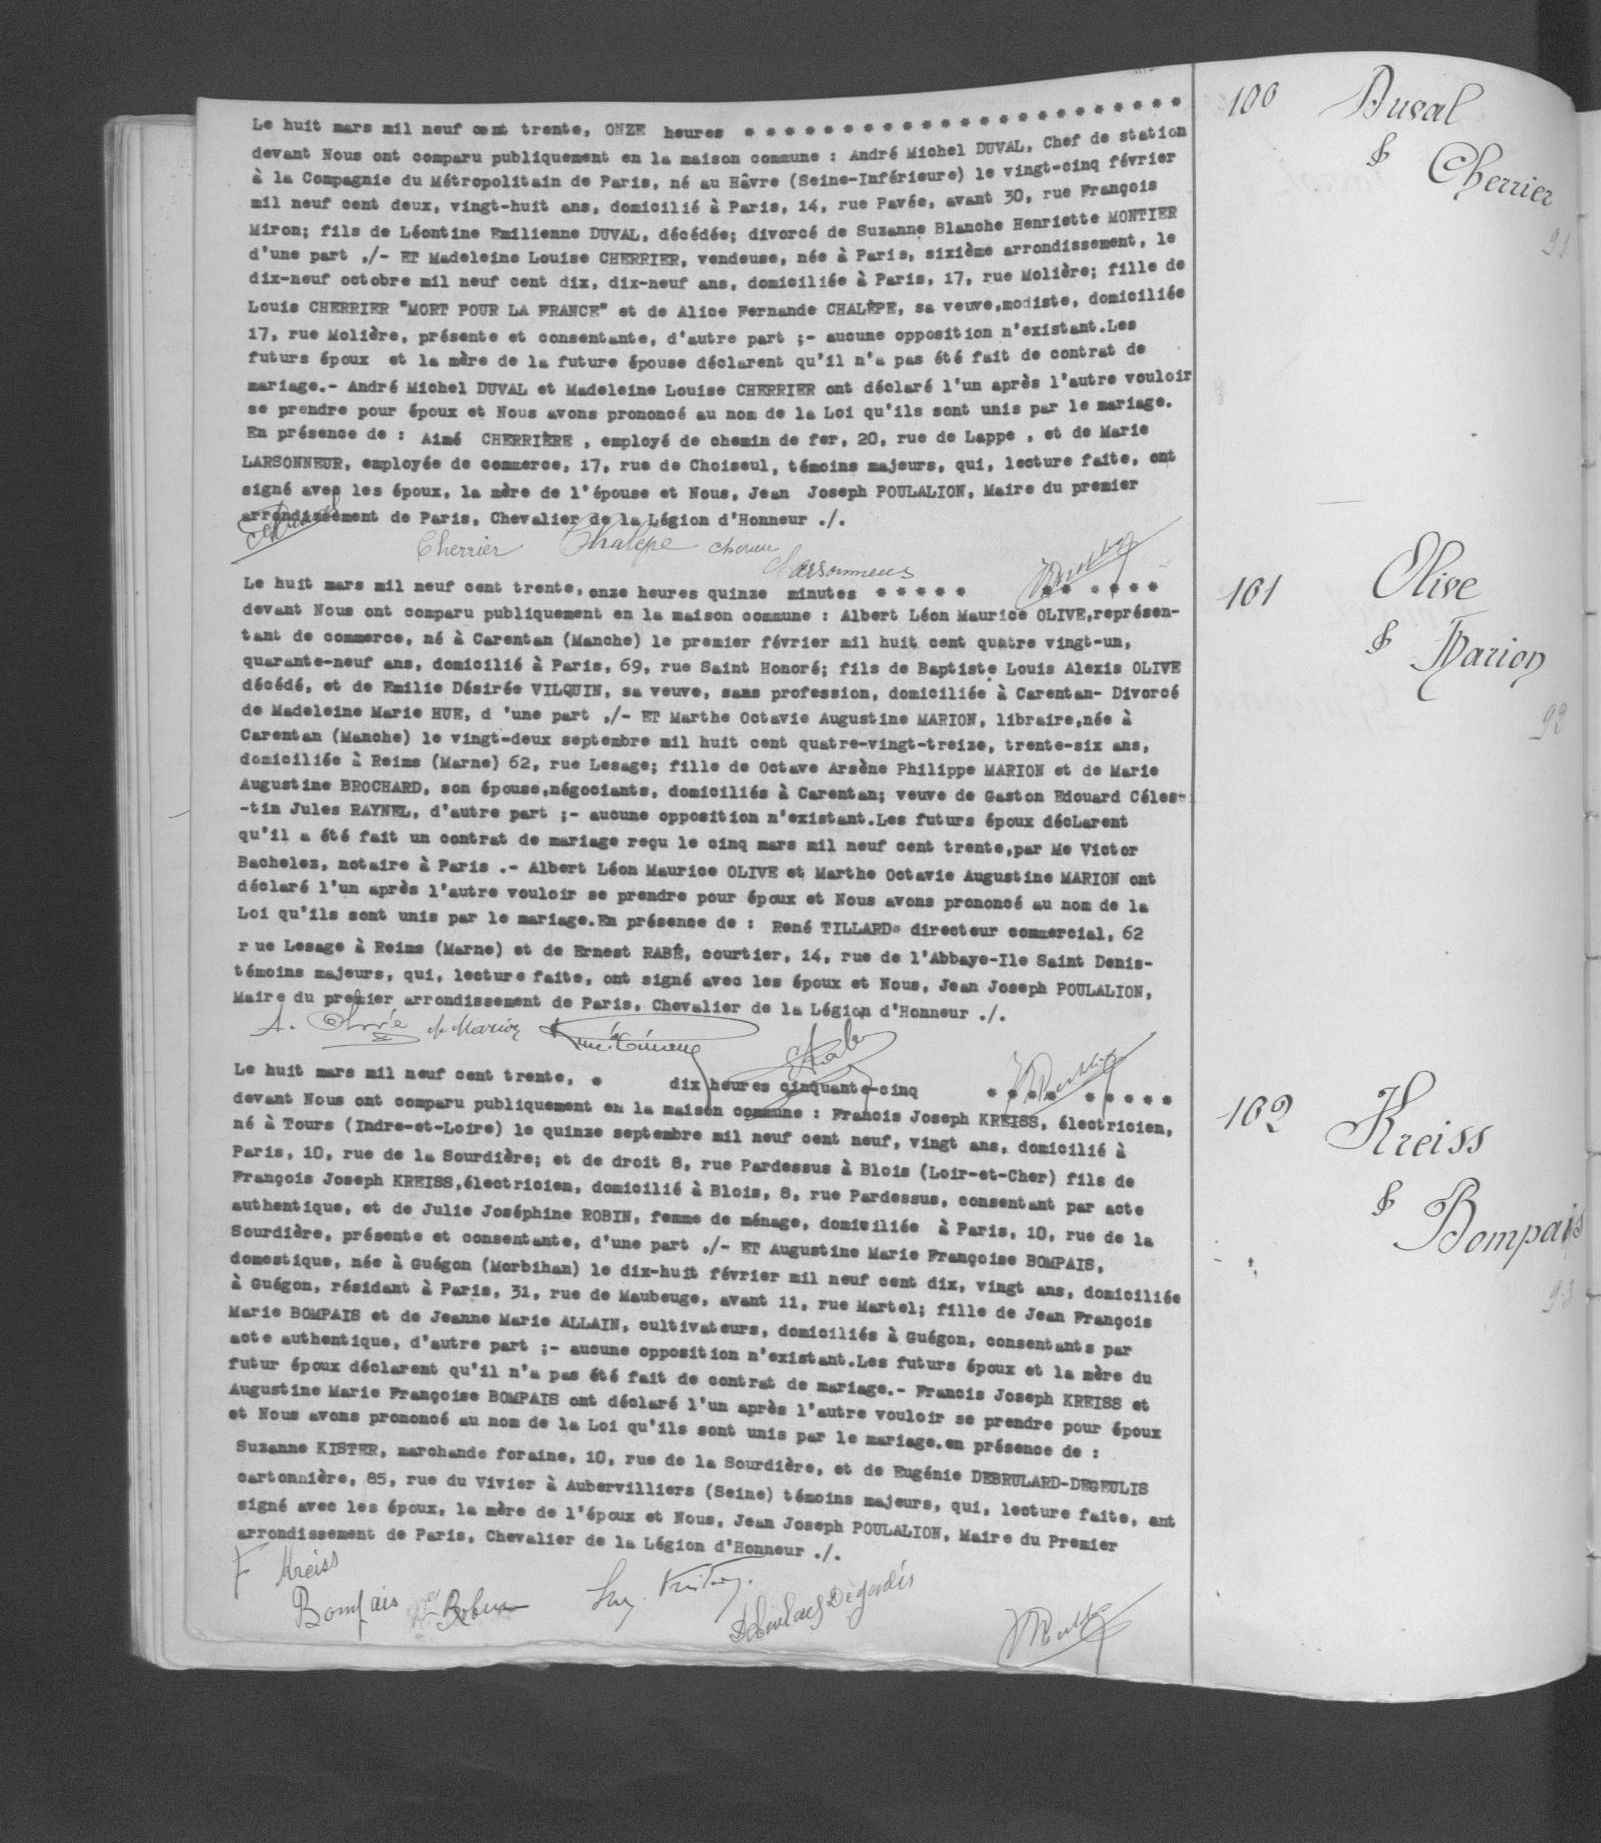Le huit mars mil neuf cent trente, onze heures ****
devant Nous ont comparu publiquement en la maison commune: André Michel DUVAL Chef de station
à la Compagnie du Métropolitain de Paris, né au Hâvre (Seine-Inférieure) le vingt-cinq février
mil neuf cent deux, vingt-huit ans, domicilié à Paris, 14, rue Pavée, avant 30, rue François
Miron; fils de Léontine Emilienne DUVAL, décédée; divorcé de Suzanne Blanche Henriette MONTIER
d'une part ,/-ET Madeleine Louise CHERRIER, vendeuse, née à Paris, sixième arronidssement, le
dix-neuf octobre mil neuf cent dix, dix-neuf ans, domiciliée à Paris, 17, rue Molière; fille de
Louis CHERRIER "MORT POUR LA FRANCE" et de Alice Fernande CHALEPE, sa veuve, modiste, domiciliée
17, rue Molière, présente et consentante, d'autre part ;-aucune opposition n'existant.Les
futurs époux et la mère de la future épouse déclarent qu'il n'a pas été fait de contrat de
mariage .-André michel DUVAL et Madeleie Louise CHERRIER ont déclaré l'un après l'autre vouloir
se prendre pour époux et Nous avons prononcé au nom de la Loi qu'ils sont unis par le mariage.
En présence de: Aimé CHERRIERE, employé de chemin de fer, 20, rue de Lappe, et de Marie
LARSONNEUR, employée de commerce, 17, rue de Choiseul, témoins majeurs, qui lecture faite, ont
signé avec les époux, la mère de l'épouse et Nous, Jean Joseph POULALION, Maire du premier
arrondissement de Paris, Chevalier de la Légion d'Honneur ./.
Le huit mars mil neuf cent trente, onze heures quinze ****
devant Nous ont comparu publiquement en la maison commune: Albert Léon Maurice OLIVE, représen-
tant de commerce, né à Carentan (Manche) le premier février mil huit cent quatre vingt-un,
quarante-neuf ans, domicilié à Paris, 69, rue Saint Honoré; fils de Baptiste Louis Alexis OLIVE
décédé, et de Emilie Désirée VILQUIN, sa veuve, sans profession, domiciliée à Carentan-Divorcé
de Madeleine Marie HUE, d'une part ,/-ET Marthe Octavie Augustine MARION, libraire, née à
Carentan (Manche) le vingt-deux septembre mil huit cent quatre-vigt-treize, trente-six ans,
domiciliée à REims (Marne) 62, rue Lesage; fille de Octave Arsène Philippe MARION et de Marie
Augustine BROCHARD, son épouse, négociante, domiciliés à Carentan; veuve de Gaston Edouard Céles-
-tin Jules RAYNEL, d'autre part ;-aucune opposition n'existant. Les futurs époux décLarent
qu'il a été fait un contrat de mariage reçu le cinq mars mil neuf cent trente, par Me Victor
Bachelez, notaire à Paris .-Albert Léon Maurice OLIVE et Marthe Octavie Augustine MARION ont
déclaré l'un après l'autre vouloir se prendre pour époux et Nous avons prononcé au nom de la
Loi qu'ils sont unis par le mariage. En présence de: René TILLARD * directeur commercial, 62
rue Lesage à Reims (Marne) et de Ernest RABE, courtier, 14, rue de l'Abbaye-Ile Saint Denis-
témoins majeurs, qui, lecture faite, ont signé avec les époux et Nous, Jean Joseph POULALION,
Maire du premier arrondissement de Paris, Chevalier de la Légion d'Honneur ./.
Le huit mars mil neuf cent trente, * dix heures cinquante-cinq ****
devant Nous ont comparu publiquement en la maison commune: Francis Joseph KREISS, électricien,
né à Tours (Indre-et-Loire) le quinze septembre mil neuf cent neuf, vingt ans, domicilié à
Paris, 10, rue de la Sourdière; et de droit 8, rue Pardessus à Blois (Loir-et-Cher) fils de
François Joseph KREISS, électricien, domicilié à Blois, 8, rue Pardessus, consentant par acte
authentique, et de Julie Joséphine ROBIN, femme de ménage, domiciliée à Paris, 10, rue de la
Sourdière, présente et consentante, d'une part ,/-ET Augustine Marie Françoise BOMPAIS,
domestique, née à Guégon (Morbihan) le dix-huit février mil neuf cent dix, vingt ans, domiciliée
à Guégon, résidant à Paris, 31, rue de Maubeuge, avant 11, rue Martel; fille de Jean François
Marie BOMPAIS et de Jeanne Marie ALLAIN, cultivateurs, domiciliés à Guégon, consentants par
acte authentique, d'autre part ;-aucune opposition n'existant. Les futurs époux et la mère du
futur époux déclarent qu'il n'a pas été fait de contrat de mariage .-Francis Joseph KREISS et
Augustine Marie Françoise BOMPAIS ont déclaré l'un après l'autre vouloir se prendre pour époux
et Nous avons prononcé au nom de la Loi qu'ils sont unis par le mariage, en présence de:
Suzanne KISTER, marchande foraine, 10, rue de la Sourdière, et de Eugénie DEBRULARD-DEGEULIS
cartonnière, 85, rue du Vivier à Aubervilliers (Seine) témoins majeurs, qui, lecture faite, ont
signé avec les époux, la mère de l'époux et Nous, Jean Joseph POULALION, Maire du Premier
arrondissement de Paris, Chevalier de la Légion d'Honneur ./.
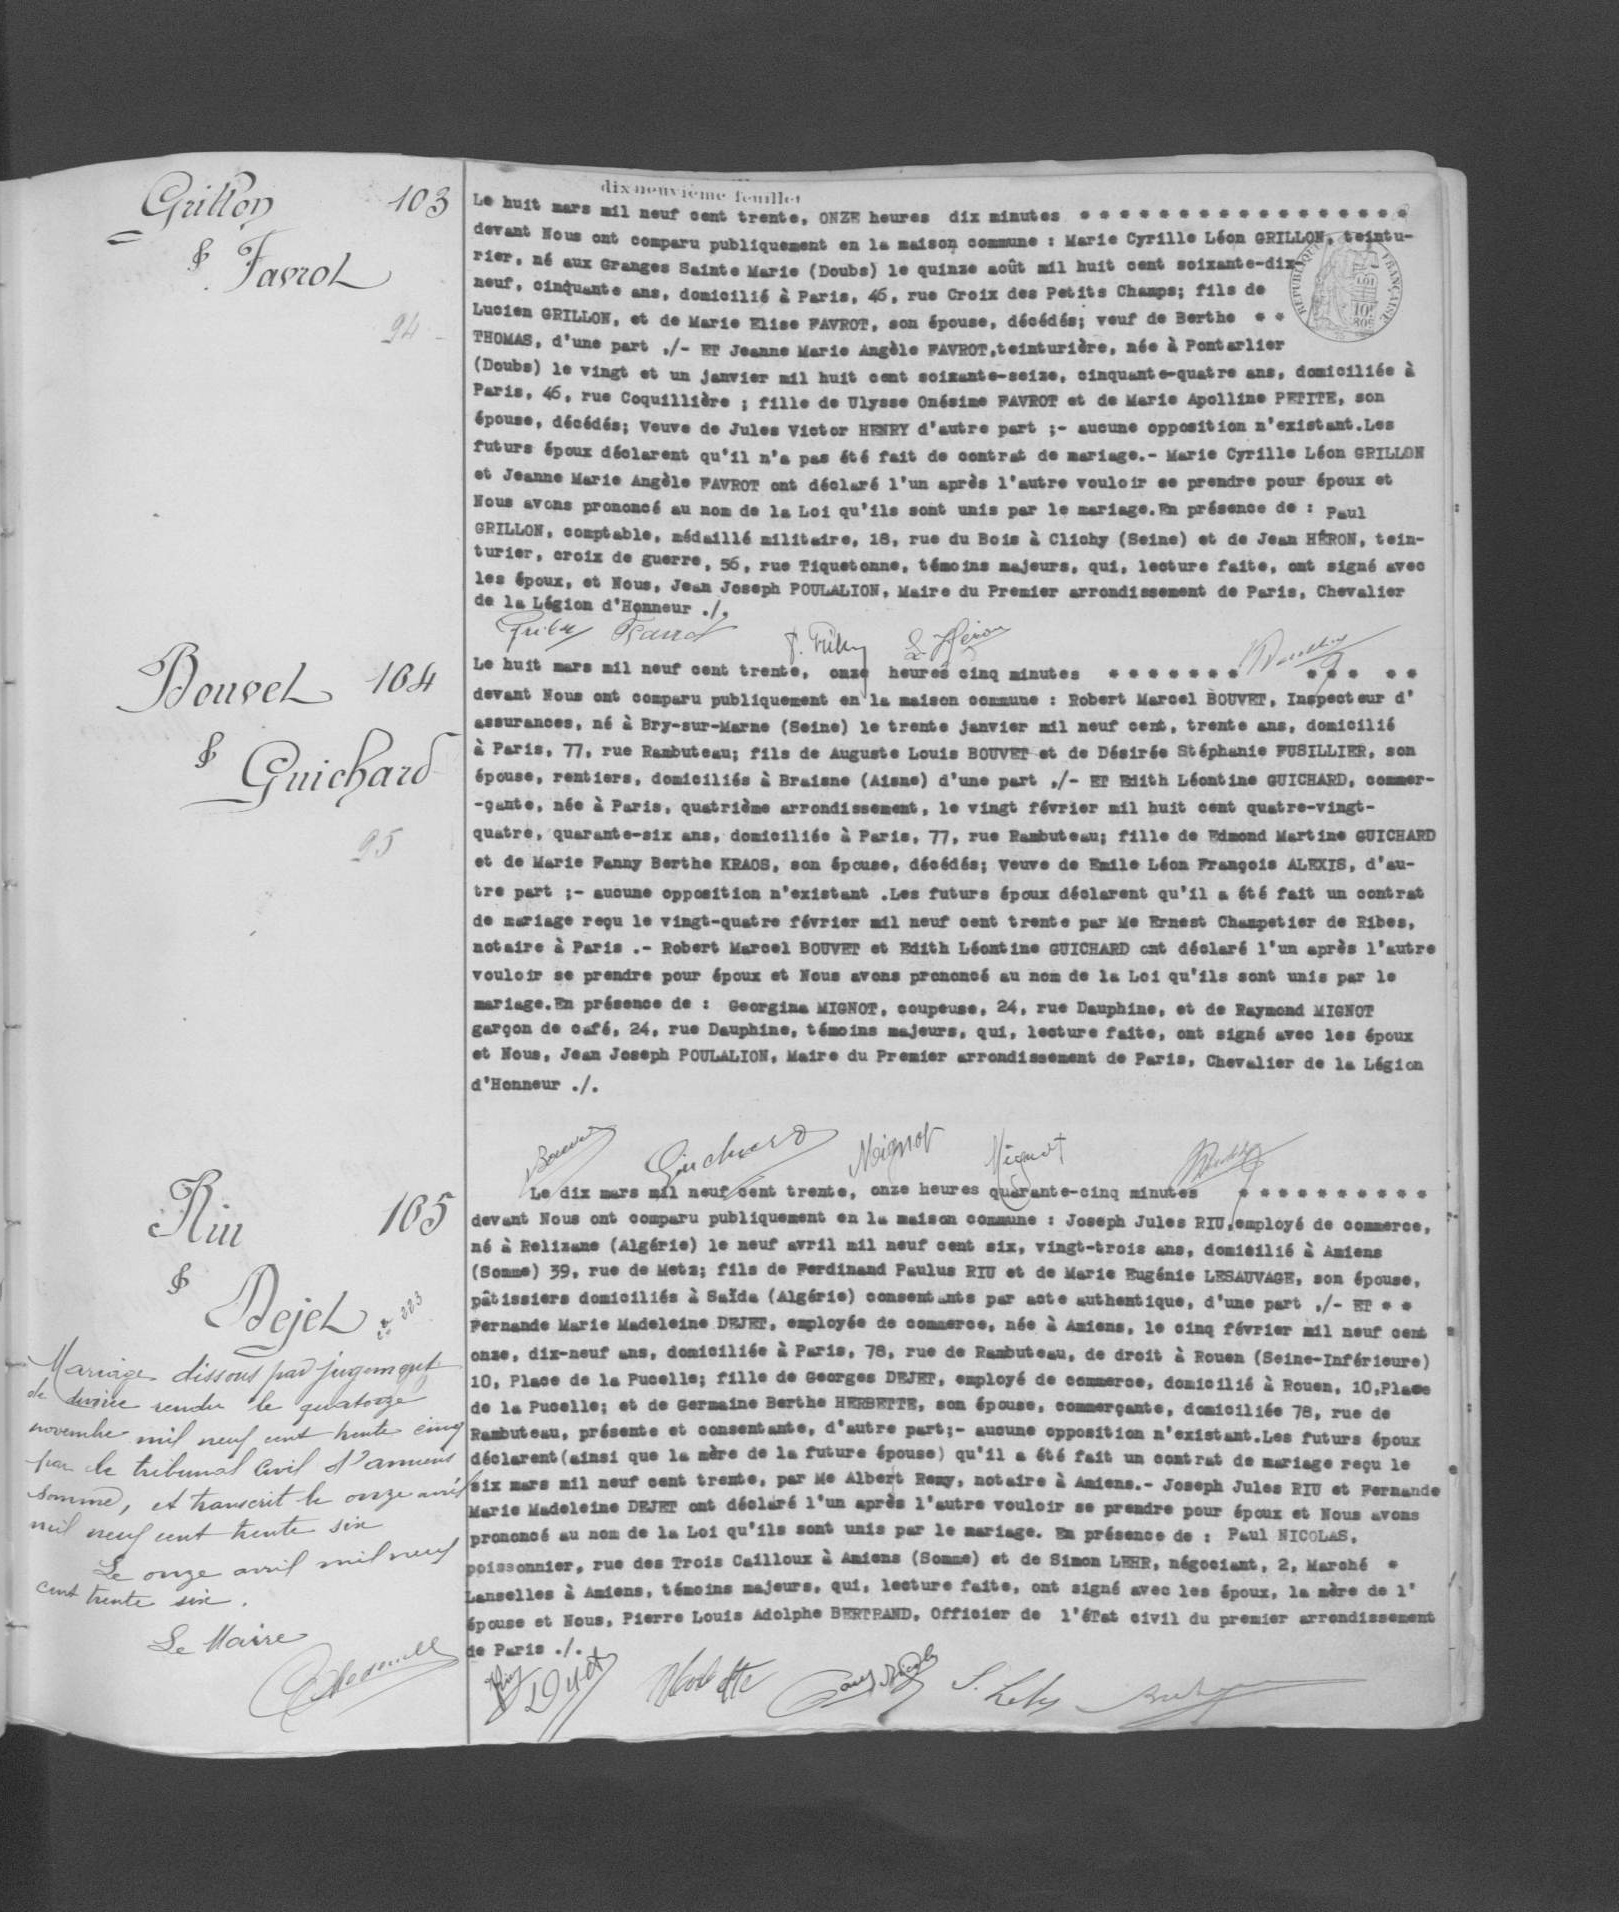Le huit mars mil neuf cent trente, ONZE heures dix minutes ****
devant Nous ont comparu publiquement en la maison commune: Marie Cyrielle Léon GRILLON, teintu-
rier, né aux Granges Sainte Marie (Doubs) le quinze août mil huit cent soixante-dix-
neuf, cinquante ans, domicilié à Paris, 46, rue Croix des Petits Champs; fils de
Lucien GRILLON, et de Marie Elise FAVROT, son épouse, décédés; veuf de Berthe * *
THOMAS, d'une part ,/-ET Jeanne Marie Angèle FAVROT, teinturière, née à Pontarlier
(Doubs) le vingt et un janvier mil huit cent soixante-seize, cinquante-quatre ans, domiciliée à
Paris, 46, rue Coquillière; fille de Ulysse Onésine FAVROT et de Marie Apolline PETITE, son
épouse, décédés; Veuve de Jules Victor HENRY d'autre part ;-aucune opposition n'existant.Les
futurs époux déclarent qu'il n'a pas été fait de contrat de mariage .-Marie Cyrille Léon GRILLON
et Jeanne Marie Angèle FAVROT, ont déclaré l'un après l'autre vouloir se prendre pour époux et
Nous avons prononcé au nom de la Loi qu'ils sont unis par le mariage. En présence de: Paul
GRILLON, comptable, médaillé militaire, 18, rue du Bois à Clichhy (Seine) et de Jean HERON, tein-
turier, croix de guerre, 56, rue Tiquetonne, témoins majeurs, qui, lecture faite, ont signé avec
les époux, et Nous, Jean Joseph POULALION, Maire du Premier arrondissement de Paris, Chevalier
de la Légion d'Honneur ./.
Le huit mars mil neuf cent trente, onze heures cinq minutes ****
devant Nous ont comparu publiquement en la maison commune: Robert Marcel BOUVE, Inspecteur d'
assurances, né à Bry-sur-Marne (Seine) le trente janvier mil neuf cent, trente ans, domicilié
à Paris, 77, rue Rambuteau; fils de Auguste Louis BOUVET et de Désirée Stéphanie FUSILLIER, son
épouse, rentiers, domiciliés à Braisne (Aisne) d'une part ,/-ET Edith Léontine GUICHARD, commer-
-çante, née à Paris, quatrième arrondissement, le vingt février mil huit cent quatre-vingt-
quatre, quarante-six ans, domiciliée à Paris, 77, rue Rambuteau; fille de Edmond Martine GUICHARD
et de Marie Fanny Berthe KRAOS, son épouse, décédés; veuve de Emile Léon, François ALEXIS, d'au-
tre part ;-aucune opposition n'existant. Les futurs époux déclarent qu'il a été fait un contrat
de mariage reçu le vingt-quatre février mil neuf cent trente par Me Ernest Chanpetier de Ribes,
notaire, à Paris .-Robert Marcel BOUVET et Edith Léontine GUICHARD ont déclaré l'un après l'autre
vouloir se prendre pour époux et Nous avons prononcé au nom de la Loi qu'ils sont unis par le
mariage. En présence de: Georgina MIGNOT, coupeuse, 24, rue Dauphine, et de Raymond MIGNOT
garçon de café, 24, rue Dauphine, témoins majeurs, qui, lecture faite, ont signé avec les époux
et Nous, Jean Joseph POULALION, Maire du Premier arrondissement de Paris, Chevalier de la Légion
d'Honneur ./.
Le dix mars mil neuf cent trente, onze heures quarante-cinq minutes ****
devant Nous ont comparu publiquement en la maison commune: Joseph Jules RIU, employé de commerce,
né à Relixane (Algérie) le neuf avril mil neuf cent six, vingt-trois ans, domicilié à Amiens
(Somme) 39, rue de Metz; fils de Ferdinand Paulus RIU et de Marie Eugénie LESAUVAGE, son épouse,
pâtissiers domiciliés à Saïda (Algérie) consentante par acte authentique, d'une part ,/-ET * *
Fernande Marie Madeleine DEJET, employée de commerce, née à Amiens, le cinq février mil neuf cent
onze, dix-neuf ans, domiciliée à Paris, 78, rue de Rambuteau, de droit à Rouen (Seine-Inférieure)
10, Place de la Pucelle; fille de Georges DEJET, employé de commerce, domicilié à Rouen, 10, Place
de la Pucelle; et de Germaine Berthe HERBETTE, son épouse, commerçante, domiciliée 78, rue de
Rambuteau, présente et consentante, d'autre part ;-aucune opposition n'existant. Les futurs époux
déclarent (ainsi que la mère de la future épouse) qu'il a été fait un contrat de mariage reçu le
six mars mil neuf cent trente, par Me Albert Remy, notaire à Amiens .-Joseph Jules RIU et Fernande
Marie Madeleine DEJET ont déclaré l'un après l'autre vouloir se prendre pour époux et Nous avons
prononcé au nom de la Loi qu'ils sont unis par le mariage. En présence de: Paul NICOLAS,
poissonnier, rue des Trois Cailloux à Amiens (Somme) et de Simon LEHR, négociant, 2, Marché *
Lanselles à Amiens, témoins majeurs, qui, lecture faite, ont signé avec les époux, la mère de l'
épouse et nous, Pierre Louis Adolphe BERTRAND, Officier de l'état civil du premier arrondissement
de Paris ./.